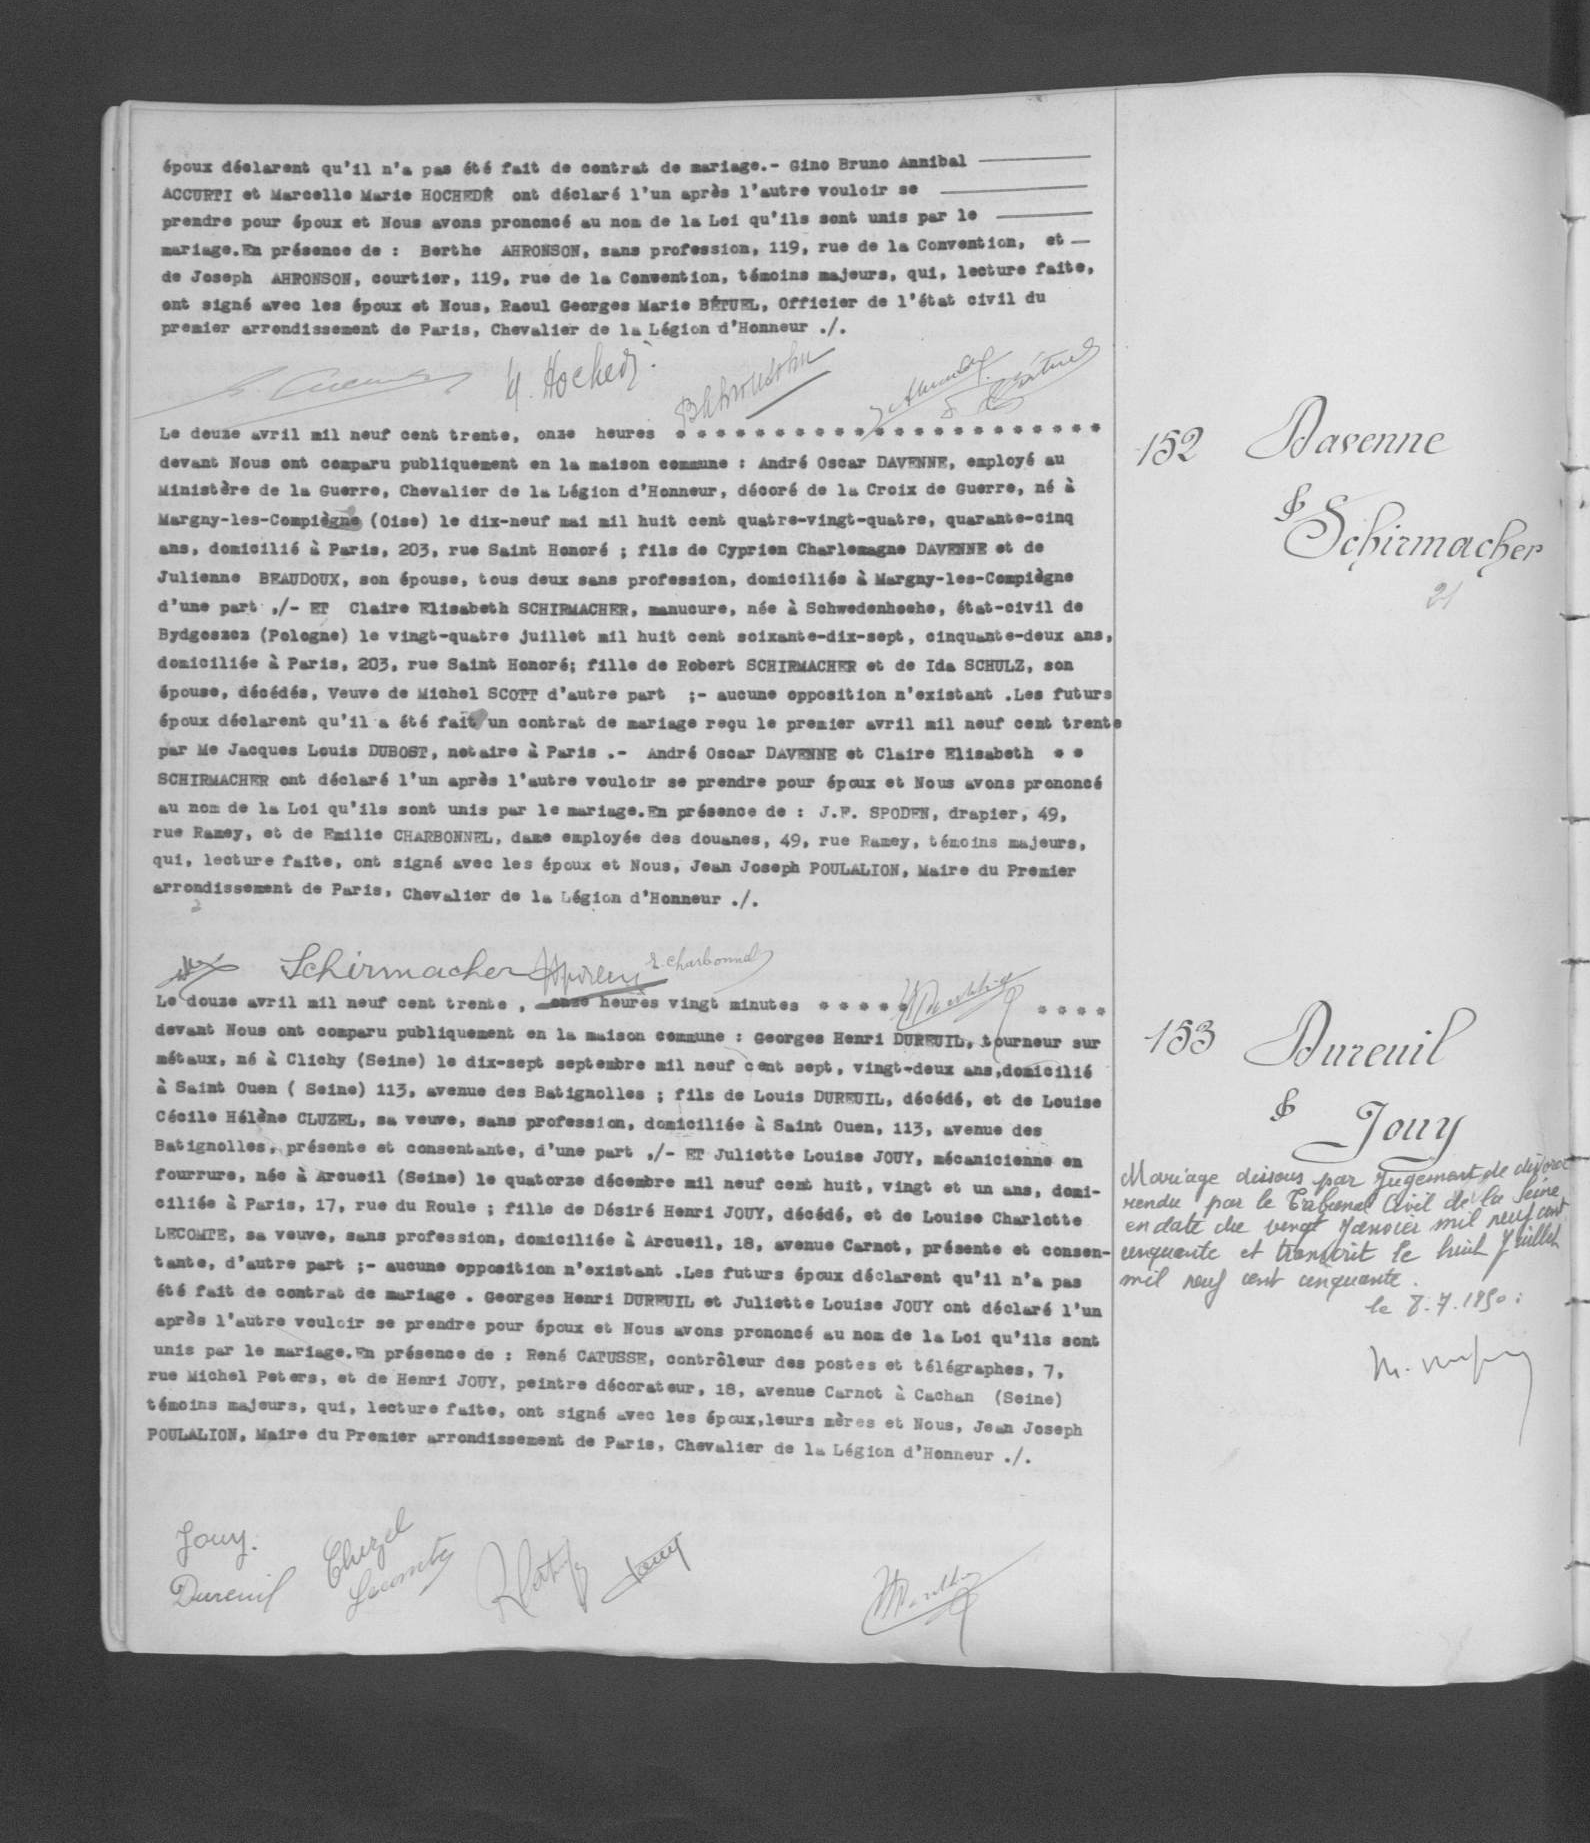époux déclarent qu'il n'a pas été fait de contrat de mariage .-Gino Bruno Annibal -
ACCURTI et Marcelle Marie HOCHERDE ont déclaré l'un après l'autre vouloir se -
prendre pour époux et Nous avons prononcé au nom de la Loi qu'ils sont unis par le -
mariage. En présence de: Berthe AHRONSON, sans profession, 119, rue de la Convention, et -
de Joseph AHRONSON, courtier, 119, rue de la Convention, témoins majeurs, qui, lecture faite,
ont signé avec les époux et Nous, Raoul Georges Marie BETUEL, Officier de l'état civil du
premier arrondissement de Paris, Chevalier de la Légion d'Honneur ./.
Le douze avril mil neuf cent trente, onze heures ****
devant nous ont comparu publiquement en la maison commune: André Oscar DAVENNE, employé au
Ministère de la Guerre, Chevalier de la Légion d'Honneur, décoré de la Croix de Guerre, né à
Margny-les-Compiègne (oise) le dix-neuf mai mil huit cent quatre-vingt-quatre, quarante-cinq
ans, domicilié à Paris, 203, rue Saint Honoré; fils de Cyprien Charlemagne DAVENNE et de
Julienne BEAUDOUX, son épouse, tous deux sans profession, domiciliés à Margny-les-Compiègne
d'une part ,/-ET Claire Elisabeth SCHIRMACHER, manucure, née à Schwedenhoehe, état-civil de
Bydgoszes (Pologne) le vingt-quatre juillet mil huit cent soixante-dix-sept, cinquante-deux ans,
domiciliée à Paris, 203, rue Saint Honoré; fille de Robert SCHIRMACHER et de Ida SCHULZ, son
épouse, décédés, Veuve de Michel SCOTT d'autre part ;-aucune opposition n'existat. Les futurs
époux déclarent qu'il a été fait un contrat de mariage reçu le premier avril mil neuf cent trente
par Me Jacques Louis DUBOST, notaire à Paris. 6 André Oscar DAVENNE et Claire Elisabeth * *
SCHIRMACHER ont déclaré l'un après l'autre vouloir se prendre pour époux et Nous avons prononcé
au nom de la Loi qu'ils sont unis par le mariage. En présence de: J. F. SPODEN, drapier, 49,
rue Ramey, et de Emilie CHARBONNEL, dame employée des douanes, 49, rue Ramey, témoins majeurs,
qui, lecture faite, ont signé avec les époux et Nous, Jean Joseph POULALION, Maire du Premier
arrondissement de Paris, Chevalier de la Légion d'Honneur ./.
Le douze avril mil neuf cent trente, onze heures vingt minutes ****
devant Nous ont comparu publiquement en la maison commune: Georges Henri DUREUIL, tourneur sur
métaux, né à Clichy (Seine) le dix-sept septembre mil neuf cent sept, vingt-deux ans, domicilié
à Saint Ouen (Seine) 113, avenue des Batignolles; fils de Louis DUREUIL, décédé, et de Louise
Cécile Hélène CLUZEL, sa veuve, sans profession, domiciliée à Saint Ouen, 113, avenue des
Batignolles, présente et consentante, d'une part ,/-ET Juliette Louise JOUY, mécanicienne en
fourrure, née à Arcueil (Seine) le quatorze décembre mil neuf cent huit, vingt et un ans, domi-
ciliée à Paris, 17, rue du Roule; fille de Désiré Henri JOUY, décédé, et de Louise Charlotte
LECOMTE, sa veuve, sans profession, domiciliée à Arcueil, 18, avenue Carnot, présente et consen-
tante, d'autre part ;-aucune opposition n'existant. Les futurs époux déclarent qu'il n'a pas
été fait de contrat de mariage. Georges Henri DUREUIL et Juliette Louise JOUY ont déclaré l'un
après l'autre vouloir se prendre pour époux et Nous avons prononcé au nom de la Loi qu'ils sont
unis par le mariage. En présence de: René CATUSSE, contrôleur des postes et télégraphes, 7,
rue Michel Peters, et de Henri JOUY, peintre décorateur, 18, avenue Carnot à Cachan (Seine)
témoins majeurs, qui, lecture faite, ont signé avec les époux, leurs mères et Nous Jean Joseph
POULALION, Maire du Premier arrondissement de Paris, Chevalier de la Légion d'Honneur ./.
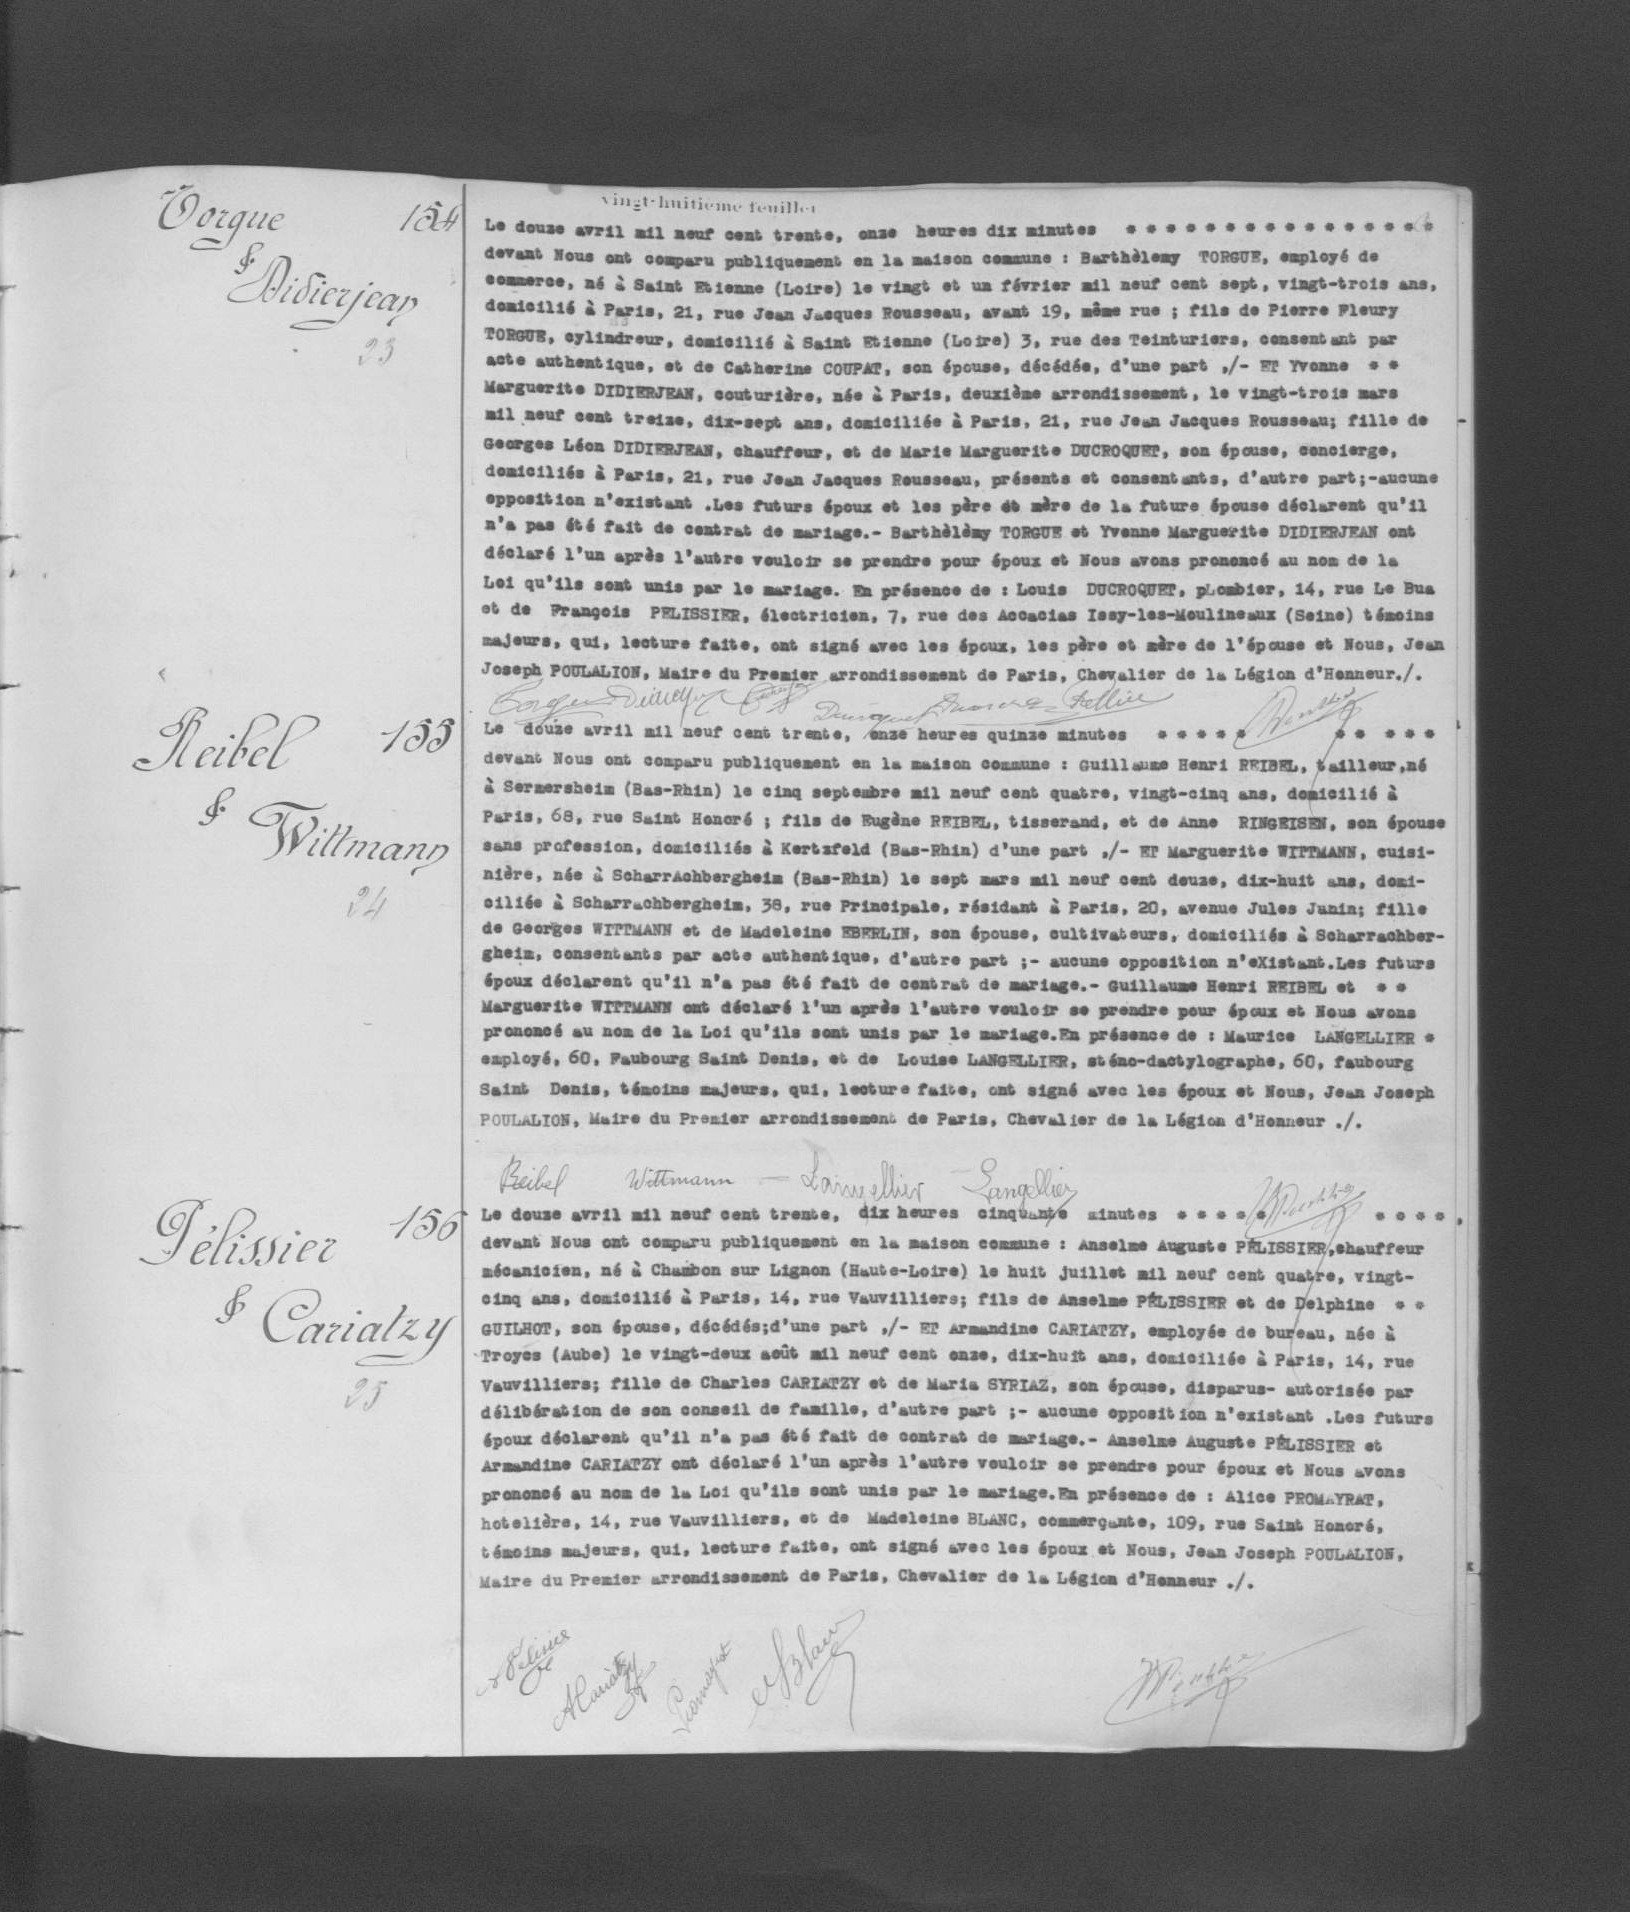Le douze avril mil neuf cent trente, onze heures dix minutes ****
devant Nous ont comparu publiquement en la maison commune: Barthèlemy TORGUE, employé de
commerce, né à Saint Etienne (Loire) le vingt et un février mil neuf cent sept, vingt-trois ans,
domicilié à Paris, 21, rue Jean Jacques Rouseau, avant 19, même rue; fils de Pierre Fleury
TORGUE, cylindreur, domicilié à Saint Etienne (Loire) 3, rue des Teinturiers, consentant par
acte authentique, et de Catherine COUPAT, son épouse, décédée, d'une part ,/-ET Yvonne * *
Marguerite DIDIERJEAN, couturière, née à Paris, deuxième arrondissement, le vingt-trois mars
mil neuf cent treize, dix-sept ans, domiciliée à paris, 21, rue Jean Jacques Rousseau; fille de
Georges Léon DIDIERJEAN, chauffeur, et de Marie Marguerite DUCROQQUET, son épouse, concierge,
domiciliés à Paris, 21, rue Jean Jacques Rousseau, présents et consentants, d'autre part ;-aucune
opposition n'existant. Les futurs époux et les père et mère de la future épouse déclarent qu'il
n'a pas été fait de contrat de mariage .-Barthèlèmy TORGUE et Yvonne Marguerite DIDIERJEAN ont
déclaré l'un après l'autre vouloir se prendre pour époux et Nous avons prononcé au nom de la
Loi qu'ils sont unis par le mariage. En présence de: Louis DUCROQUET, pLombier, 14, rue Le Bua
et de François PELISSIER, électricien, 7, rue des Accacias Issy-les-Moulineaux (Seine) témoins
majeurs, qui, lecture faite, ont signé avec les époux, les père et mère de l'épouse et Nous, Jean
Joseph POULALION, Maire du Premier arrondissement de Paris, Chevalier de la Légion d'Honneur ./.
Le douze avril mil neuf cent trente, onze heures quinze minutes ****
devant Nous ont comparu publiquement en la maison commune: Guillaume Henri REIBEL, tailleur, né
à Sermersheim (Bas-Rhin) le cinq septembre mil neuf cent quatre, vingt-cinq ans, domicilié à
Paris, 68, rue Saint Honoré; fils de Eugène REIBEL, tisserand, et de Anne RINGEISEN, son épouse
sans profession, domiciliés à Kertzfeld (Bas-Rhin) d'une part ,/-ET Marguerite WITTMANN, cuisi-
nière, née à Scharrachbergheim (Bas-Rhin) le sept mars mil neuf cent douze, dix-huit ans, domi-
ciliée à Scharrachbergheim, 38, rue Principale, résidant à Paris, 20, avenue Jules Junin; fille
de Georges WITTMANN et de Madeleine EBERLIN, son épouse, cultivateurs, domiciliés à Scharrachber-
gheim, consentants par acte authentique, d'autre part ;-aucune opposition n'eXistant. Les futurs
époux déclarent qu'il n'a pas été fait de contrat de mariage .-Guillaume Henri REIBEL et * *
Marguerite WITTMANN ont déclaré l'un après l'autre vouloir se prendre pour époux et Nous avons
prononcé au nom de la Loi qu'ils sont unis par le mariage. En présence de: Maurice LANGELLIER *
employé, 60, Faubourg Saint Denis, et de Louise LANGELLIER, sténo-dactylographe, 60, faubourg
Saint Denis, témoins majeurs, qui, lecture faite, ont signé avec les époux et Nous, Jean Joseph
POULALION, Maire du Premier arrondissement de Paris, Chevalier de la Légion d'Honneur ./.
Le douze avril mil neuf cent trente, dix heures cinquante minutes ****
devant Nous ont comparu publiquement en la maison commune: Anselme Auguste PELISSIER, chauffeur
mécanicien, né à Chambon sur Lignon (Haute-Loire) le huit juillet mil neuf cent quatre, vingt-
cinq ans, domicilié à Paris, 14, rue Vauvilliers; fils de Anselme PELISSIER et de Delphine * *
GUILHOT, son épouse, décédés; d'une part ,/-ET Armandine CARIATZY, employée de bureau, née à
Troyes (Aube) le vingt-deux août mil neuf cent onze, dix-huit ans, domiciliée à Paris, 14, rue
Vauvillier; fille de Charles CARIATZY et de Maria SYRIAZ, son épouse, disparus-autorisée par
délibération de son conseil de famille, d'autre part ;-aucune opposition n'existant. Les futurs
époux déclarent qu'il n'a pas été fait de contrat de mariage .-Anselme Auguste PELISSIER et
Armandine CARIATZY ont déclaré l'un après l'autre vouloir se prendre pour époux et Nous avons
prononcé au nom de la Loi qu'ils sont unis par le mariage. En présence de: Alice PROMAYRAT,
hotelière, 14, rue Vauvilliers, et de Madeleine BLANC, commerçante, 109, rue Saint Honoré,
témoins majeurs, qui, lecture faite, ont signé avec les époux et Nous, Jean Joseph POULALION,
Maire du Premier arrondisement de Paris, Chevalier de la Légion d'Honneur ./.